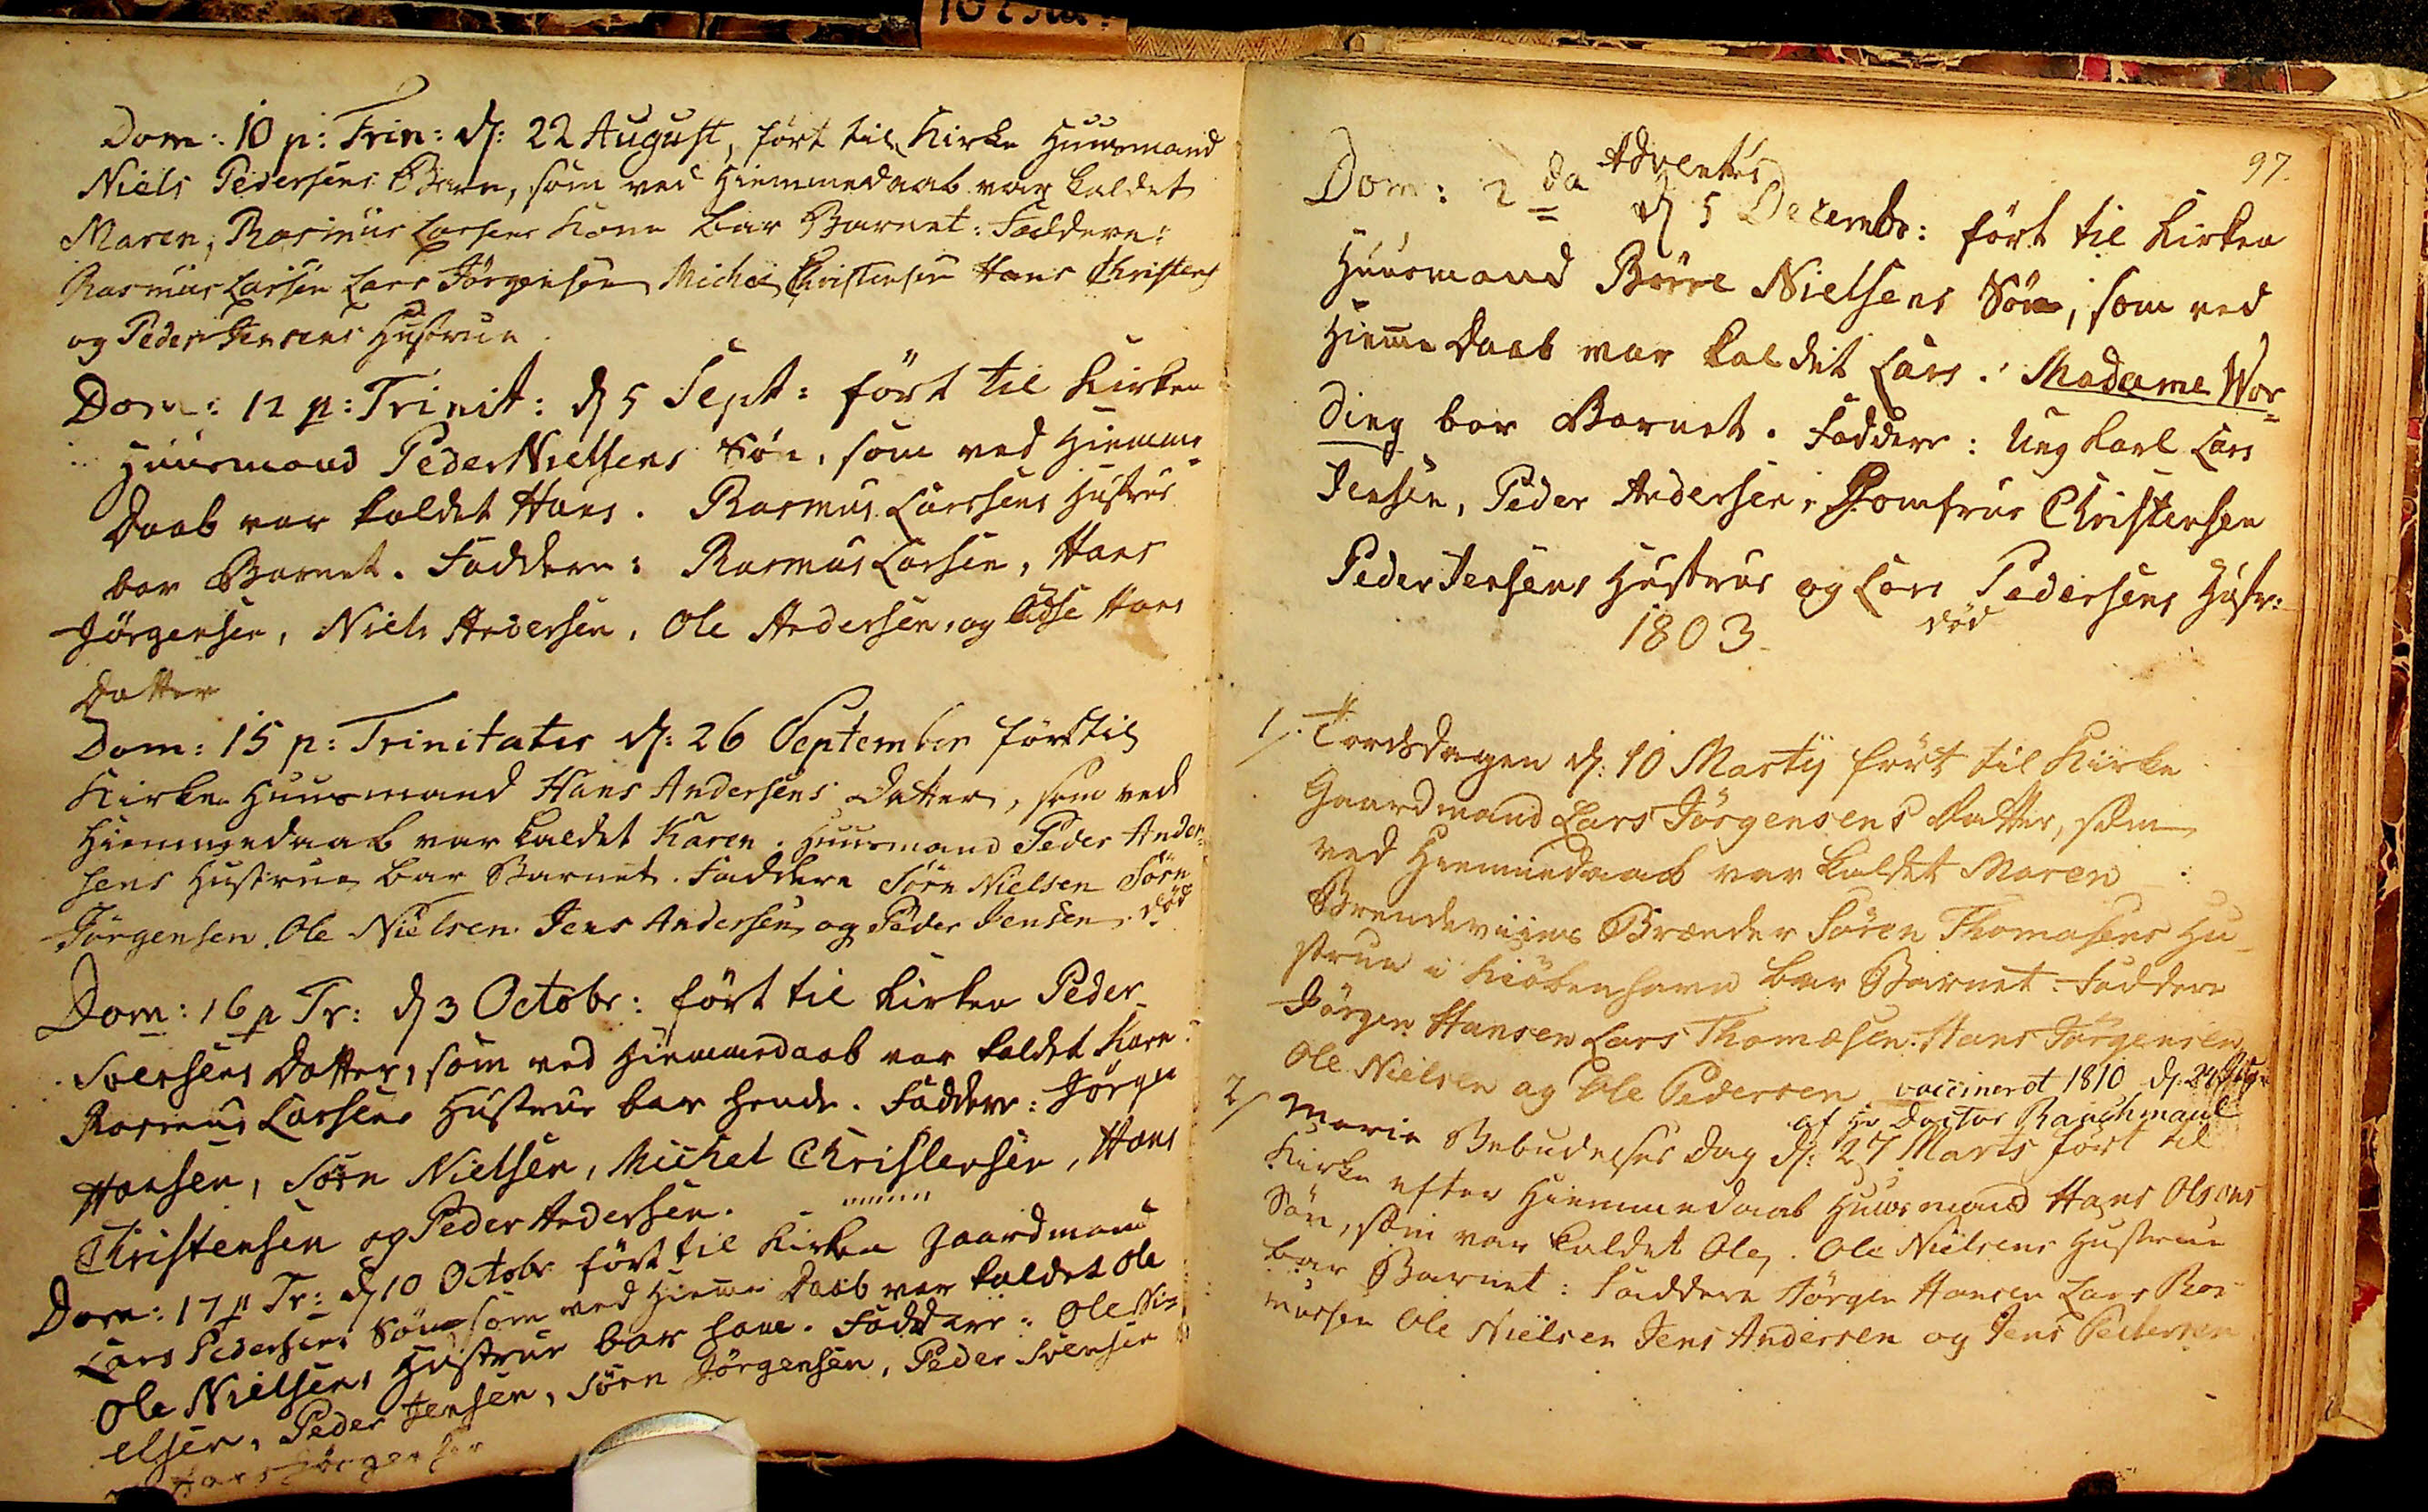Dom: 10 p: Trin: d: 22 August, ført til Kirken Huusmand
Niels Pedersens Barn, som ved Hiemmedaab var kaldet
Maren, Rasmus Larsens Kone bar Barnet: Faddere:
Rasmus Larsen Lars Jørgensen Michel Christensen Hans Christens
og Peder Jensens Hustrue.
Dom: 12 p: Trinit: d 5 Sept: ført til Kirken
Huusmand Peder Nielsens Søn, som ved Hiemme¬
Daab var kaldet Hans. Rasmus Larssens Hustrue
bar Barnet. Faddere: Rasmus Larsen, Hans
Jørgensen, Niels Andersen, Ole Andersen, og Cidse Hans
Datter
Dom: 15 p: Trinitatis d: 26 September førttil
Kirken Huusmand Hans Andersens Datter, som ved
Hiemmedaab var kaldet Karen. Huusmand Peder Ander¬
sens Hustrue bar Barnet. Faddere Sørn Nielsen Sørn
Jørgensen, Ole Nielsen, Jens Andersen, og Peder Jensen. død
Dom: 16 p Tr: d 3 Octobr: ført til Kirken Peder
Svensens Datter, som ved Hiemmedaab var kaldet Karn.
Rasmus Larsens Hustrue bar hende. Faddere: Jørgen
Hansen, Sørn Nielsen, Michel Christensen, Hans
Christensen og Peder Andersen.
Dom: 17 p Tr: d 10 Octobr. ført til Kirken Gaardmand
Lars Pedersens Søn, som ved Hiemme Daab var kaldet Ole
Ole Nielsens Hustrue bar ham. Faddere: Ole Ni¬
elsen, Peder Jensen, Sørn Jørgensen, Peder Svensen
og Hans Jørgensen
97.
Adventus    
Dom: 2da d 5 Decembr: ført til Kirken
Huusmand Børre Nielsens Søn, som ved
Hiemme Daab var kaldet Lars. Madame Wor¬
ding bar Barnet. Faddere: Ungkarl Lars
Jensen, Peder Andersen, Jomfrue Christensen
Peder Jensens Hustrue og Lars Pedersens Hustr:
død
1803.
1/ Torsdagen d: 10 Martij ført til Kirke
Gaardmand Lars Jørgensens Datter, som
ved Hiemmedaab var kaldet Maren
Brendeviins Brænder Søren Thomasens Hu¬
strue i Kiøbenhavn bar Barnet. Faddere
Jørgen Hansen Lars Thomæsen. Hans Jørgensen
Ole Nielsen og Ole Pedersen. vaccineret 1810 d: 24 Augu
af Hr Doctor Rauchmaul
2/ Maria Bebudelses Dag d. 27 Marts ført til
Kirke efter Hiemmedaab Huusmand Hans Olsons
Søn, som var kaldet Ole. Ole Nielsens Hustrue
bar Barnet: Faddere. Jørgen Hansen Lars Ras¬
mussen Ole Nielsen Jens Andersen og Jens Peitersen
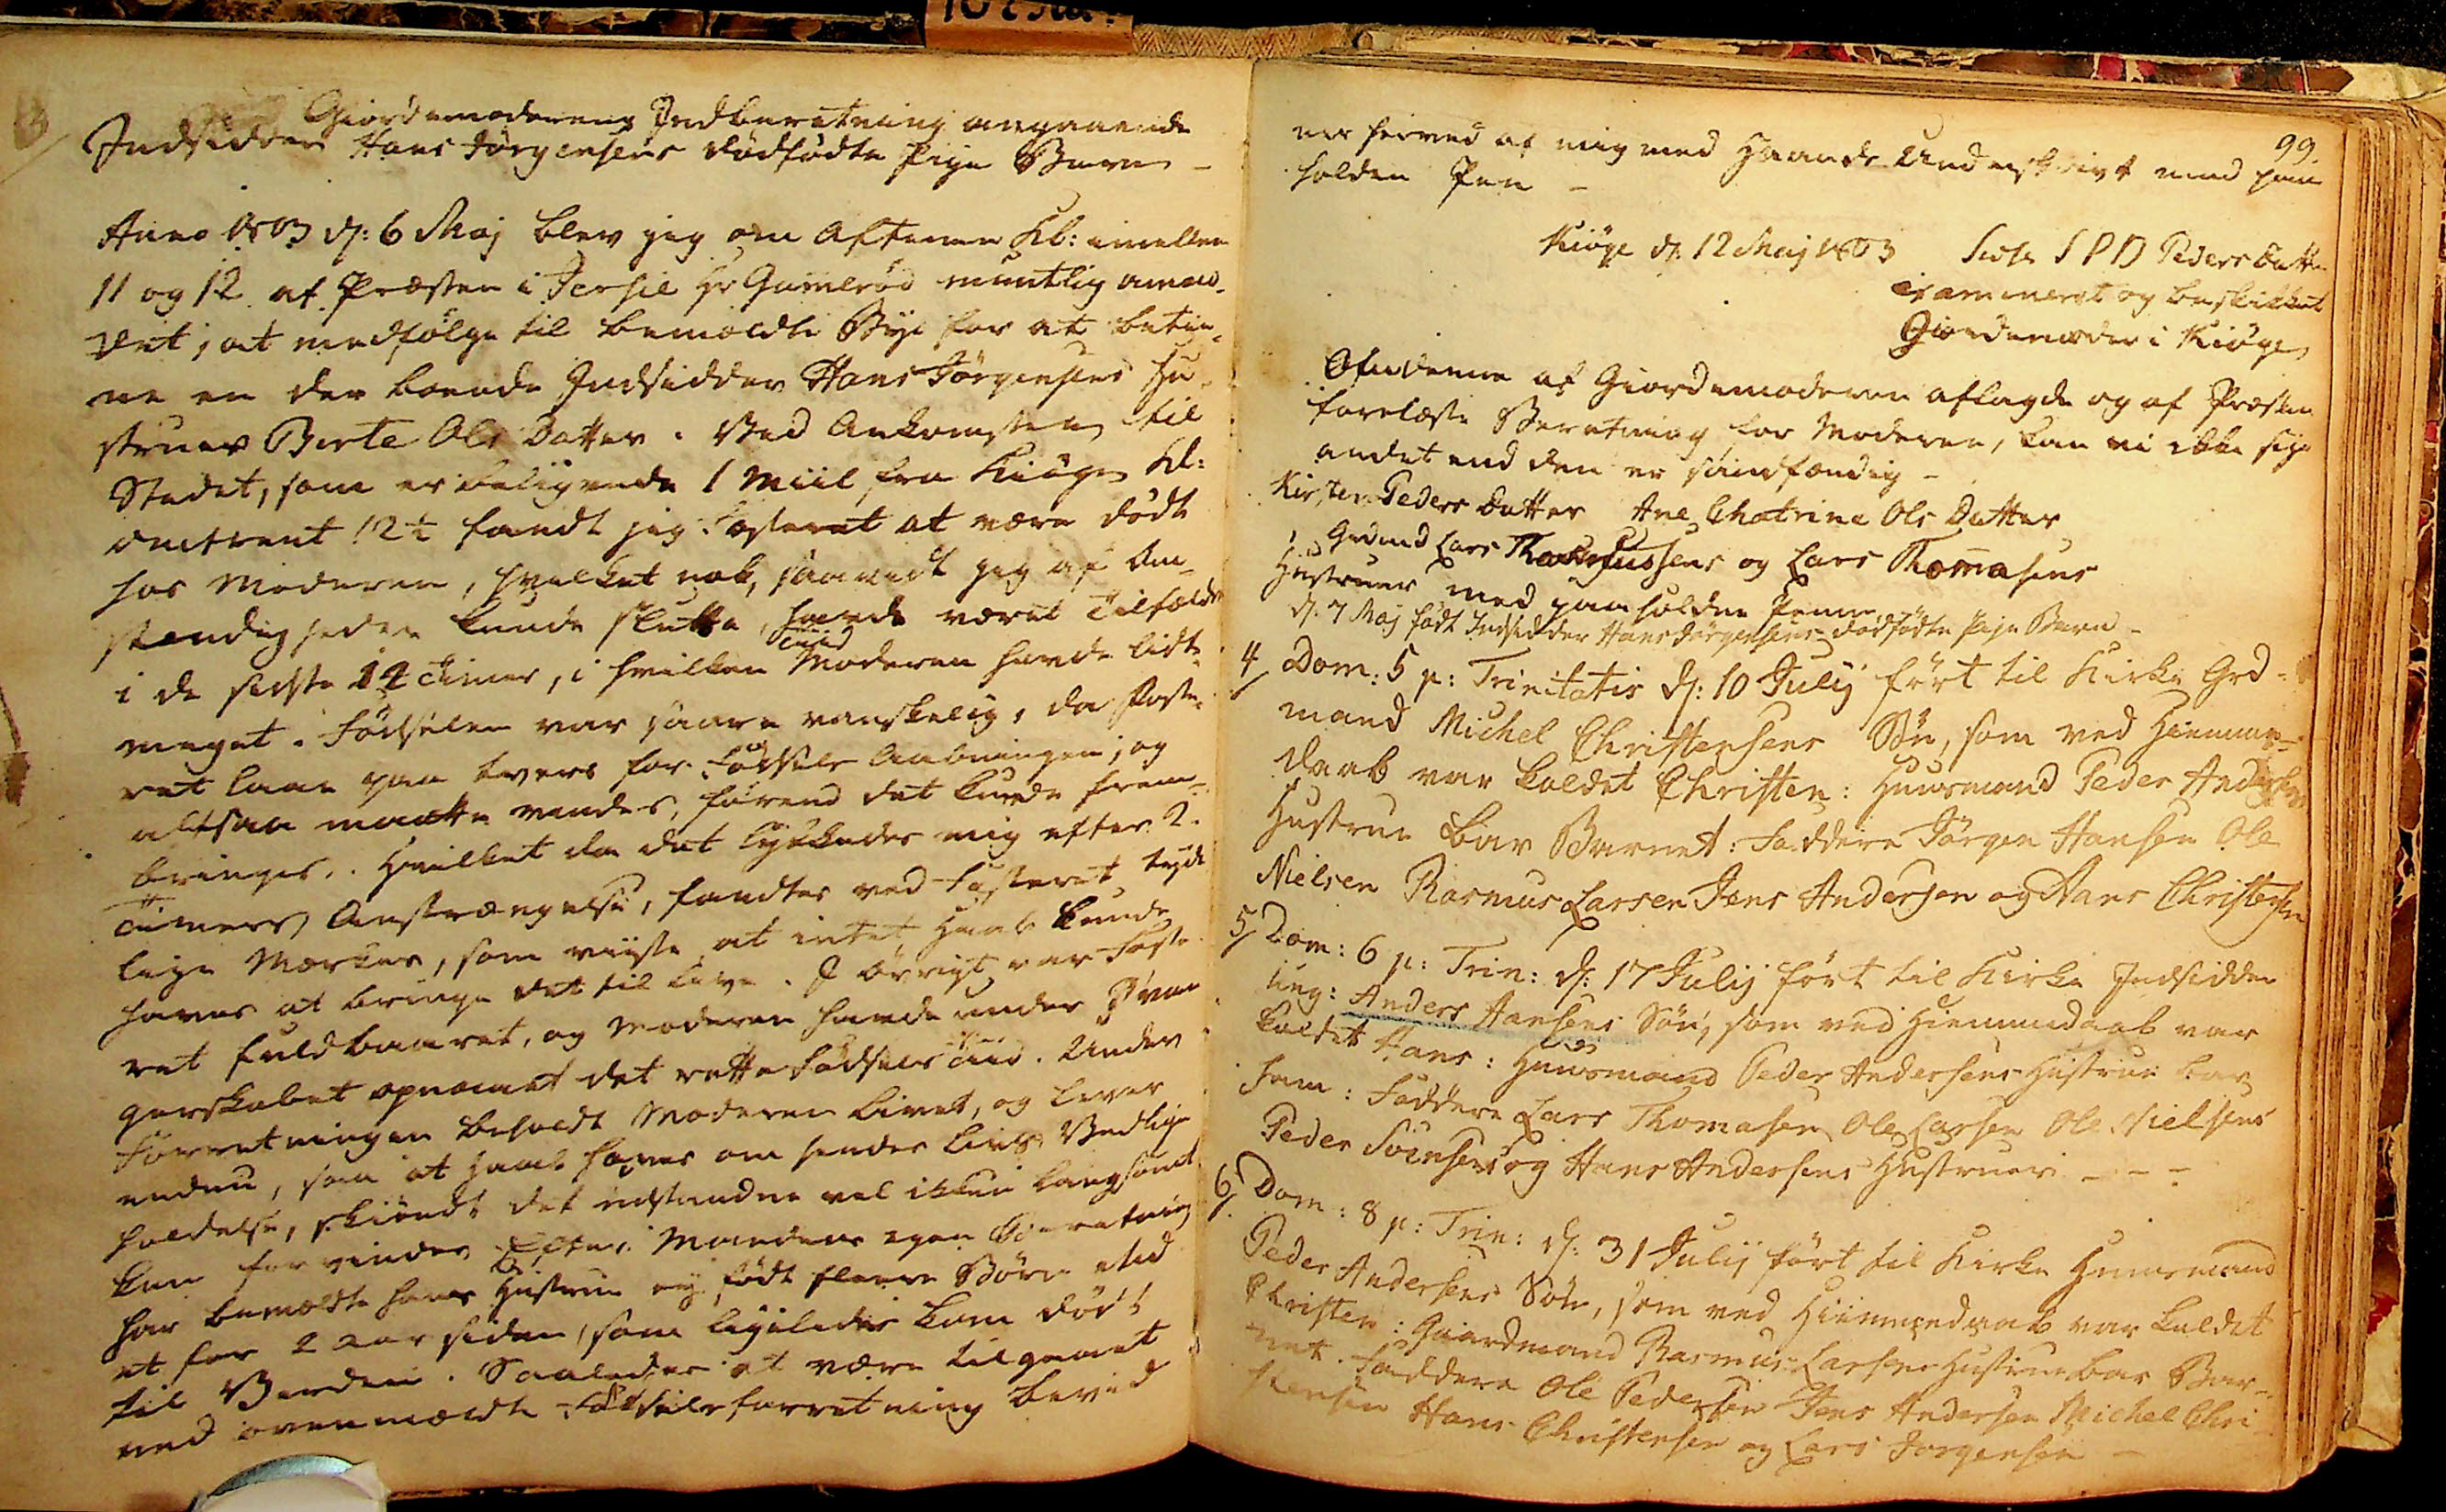3/ Giordemoderens Indberetniing angaaende
Indsidder Hans Jørgensens dødfødte Pige Barn
Anno 1803 d: 6 Maj blev jeg om Aftenen Kl: imellem
11 og 12 af Præsten i Jersie Hr Gamerød muntlig  an##¬
##let, at medfølge til bemældte Bye for at betie¬
ne en der boende Indsidder Hans Jørgensens Hu¬
strues Berte Ols Datter. Ved Ankomsten til
Stedet, som er beligende 1 Miil fra Kiøge kl:
omtrent 12½ fandt jeg Fosteret at være dødt
hos Moderen, hvilket nok, saavidt jeg af Om¬
stændigheden kunde slutte, havde været Tilfældet
Tiid
i de sidste 14 Timer, i hvilken Moderen havde lidt
meget. Fødselen var saare vanskelig, da Foste¬
ret laae paa tvers for Fødsels Aabningen; og
altsaa maatte vendes, førend det kunde frem¬
bringes, hvilket da det lykkedes mig efter 2.
Timers Anstrængelse, fandtes ved Fosteret tyde
lige Mærker, som viiste at intet havde kunde
haves at bringe det til Live. I øvrigt var foste
ret fuldbaaret, og Moderen havde under Svan
gerskabet opnaaret det rette fødsels Tiid. Under
Forretningen beholdt Moderen Livet, og lever
endnu, saa at haab haves om hendes Livs Vedlige
holdelse, skiøndt det udstandne vel ikkun langsomt
kan forvindes. Efter Mandens egen Beretning
har bemældte hans Hustrue eij født flere Børn end##
et for 2 Aar siden, som ligeledes kom dødt
til Verden. Saaledes at være tilgaaet
ved ovenmældte Fødselsforretning bevid
99.
nes herved af mig med Haands Underskrivt med paa
holden Pen
Kiøge d. 12 Maj 1803 Sidse SPD Pedersdatter
Exameneret og beskikket
Giordemoder i Kiøge,
Af denne af Giordemoderen aflagde og af Præsten
forelæste Beretning for Moderen, kan vi ikke sige
andet end den er sandfærdig -
Kirsten Peders Datter Ane Chatrine Ols Datter
Grdmd Lars Rasmussens og Lars Thommasens
Hustruer med paaholdne Penne
d: 7 Maj født Indsidder Hans Jørgensens dødfødte Pige Barn
4/ Dom: 5 p: Trinitatis d: 10 Julij ført til Kirke Grd¬
mand Michel Christensens Søn, som ved Hiemme¬
daab var kaldet Christen: Huusmand Peder Andersens
Hustrue bar Barnet: Faddere Jørgen Hansen Ole
Nielsen Rasmus Larsen Jens Andersen og Hans Christensen
5/ Dom: 6 p: Trin: d: 17 Julij ført til Kirke Indsidder
Ung: Anders Hansens Søn, som ved Hiemmedaab var
kaldet Hans: Huusmand Peder Andersens Hustrue bar
ham: Faddere Lars Thomasen Ole Larsen Ole Nielsens
Peder Svensens og Hans Andersens Hustruer - - -
6/ Dom: 8 p: Trin: d: 31 Julij ført til Kirke Huusmand
Peder Andersens Søn, som ved Hiemmedaab var kaldet
Christen: Gaardmand Rasmus Larsens Hustrue bar Bar¬
net. Faddere Ole Pedersen Jens Andersen Michel Chri¬
stensen Hans Christensen og Lars Jorgensen